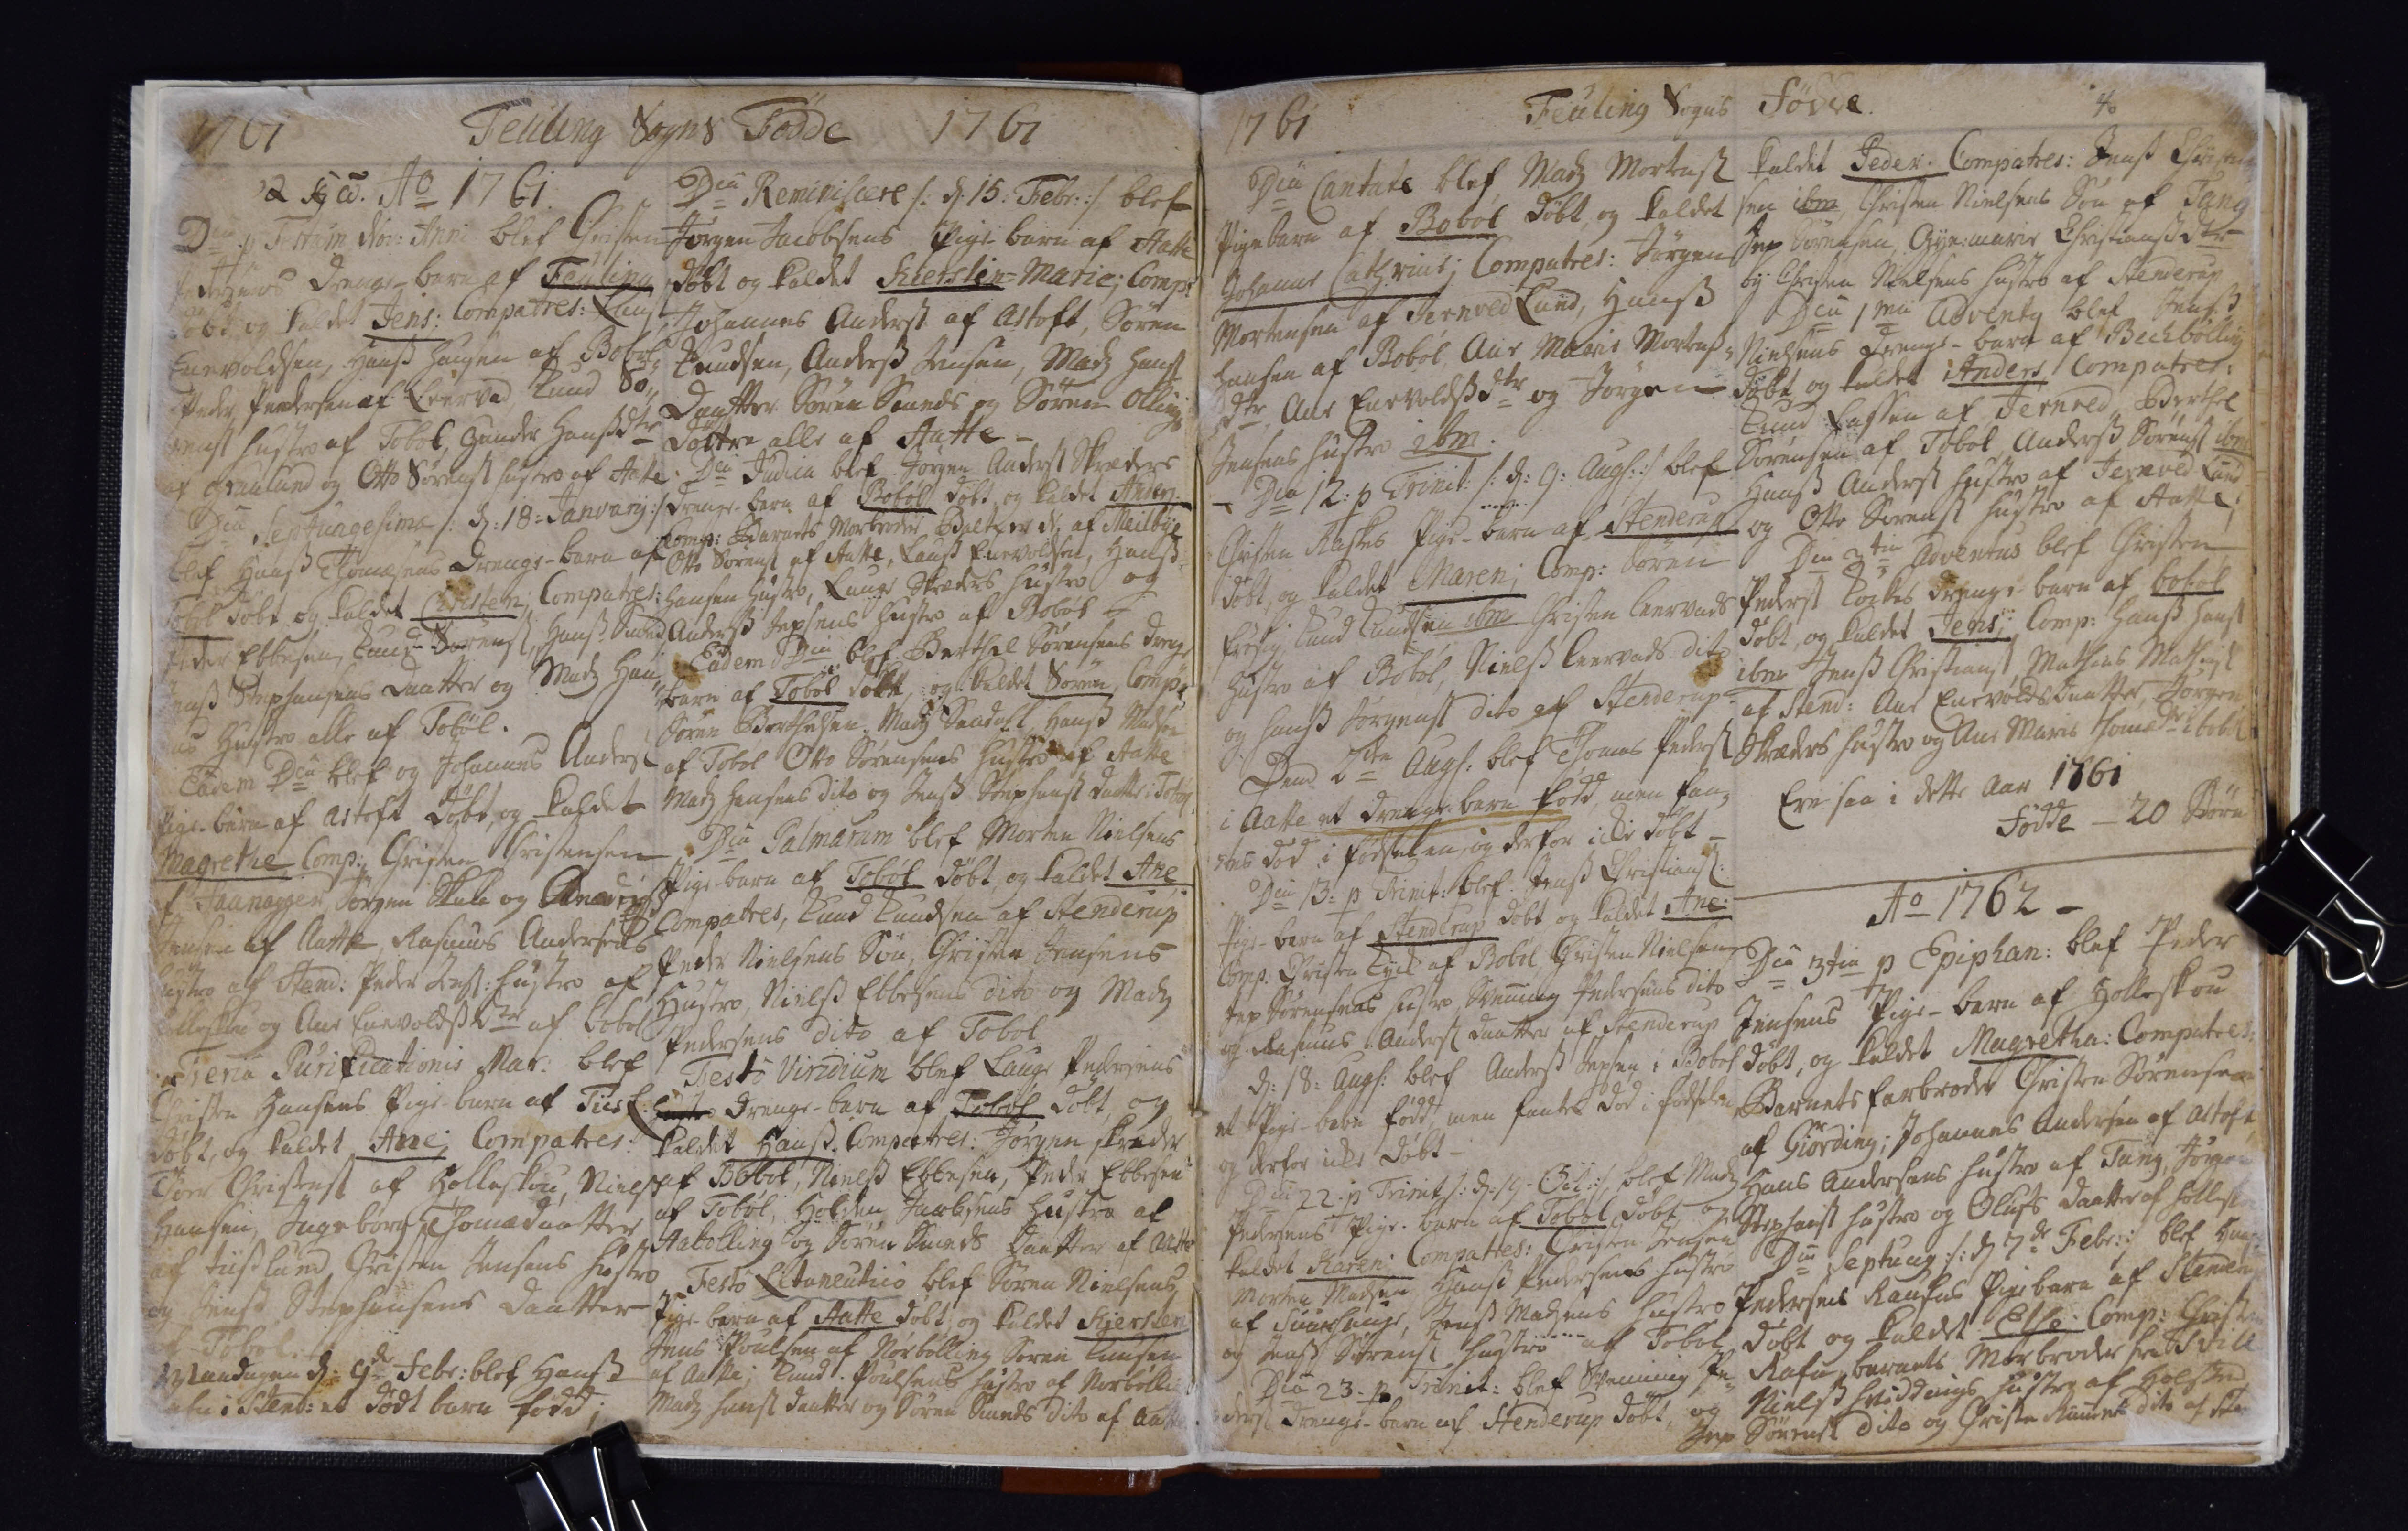1761
Feuling Sogns Fødde
##2 ##. Ao 1761
Dca p Festum Nov: Anni blef Christen
Pedersens Drenge-barn af Feuling
døbt og kaldet Jens; Compatres: Lau##
Enevoldsen, Hans Hansen af Bobøl
Peder Pedersen af Leervad, Knud Sø¬
sens Hustro af Tobøl, Gunder Hanssdtr
af Graulund og Otte Sørens Hustro af Aatte
Dca Septuagesima /: d: 18: Januarij :/
blef Hans Thomæsens Drenge-barn af
Tobøl døbt og kaldet Christen; Compatres:
Peder Ebbesøn, Knud Sørens Hans Smed
Jens Stephansens Daatter og Madz Han¬
sens Hustro alle af Tobøl.
Eodem Dca blef og Johannes Anders
Pige-barn af Aatoft døbt, og kaldet
Magrethe Comp:, Christen Christensen
af Faanagger Jørgen Skule og Anderss
Jensen af Aatte, Rasmus Andersens
Hustror af Stend: Peder Jens: Hustro af
Hollesakou og Ane Enevoldssdtr af bobøl
Feria Purificationis Mar: blef
Christen Hansens Pige-barn af Tiisl:
døbt, og kaldet Ane; Compatres:
Thoer Christens af Holleskou, Nielss
Hansen, Ingeborg Thomasdaatter
af Tiislund, Christen Jensens Hustro
og Jenss Stephansens Daatter,
af Tobøl
Mandagen d: 9de Febr: blef Hanss
Rafn i Stend: et dødt barn fødd;
1761
Dca Reminiscere /: d 15 Febr: :/ blef
Jørgen Jacobsens Pige-barn af Aatte
døbt og kaldet Kiersten=Marie; Comp:
Johannes Anders af Astoft, Søren
Knudsen, Anders Jensen, Madz Hans
Daatter. Søren Smeds og Søren Ollings
Døttre alle af Aatte
Dca Judica blef Jørgen Anders Skræders
Drenge-barn af Bobøl døbt, og kaldet Anders
Comp: Barnets Morbroder Baltzer N. af Meilbye
Otte Sørens af Aatte, Laus Envoldsen, Hanss
Hansen Hustro, Lauge Skræders Hustro og
Anderss Jepsens Hustro af Bobøl
Eadem Dca blef Berthel Sørensens Drenge¬
Barn af Tobøl døbt, og kaldet Søren, Comp:
Søren Berthelsen, Madz Sandahl, Hanss Madsen
af Tobøl Otto Sørrensens Hustro af Aatte
Matz Hansens dito og Jenss Stephans Daatter i Tobøl
Dca Palmarum blef Morten Nielsens
Pige-barn af Tobøl døbt, og kaldet Ane
Compatres, Knud Knudsen af Stenderup
Peder Nielsens Søn, Christen Jensens
Hustro, Nielss Ebbesens dito og Madz
Pedersens dito af Tobøl
Festo Viridium blef Lauge Pedersens
Hustro Drenge-barn af Tobøl døbt, og
kaldet Hanss, Compatres: Jørgen Skræder
af Bobøl, Nielss Ebbesen, Peder Ebbesen
af Tobøl, Holden Jacobsens Hustro af
Aabølling og Søren Smeds Daatter af Aatte
Festo Litaneutico blef Søren Nielsens
Pige barn af Aatte døbt, og kaldet Kiersten
Jens Poulsen af Nørbølling Søren Knudsen
af Aatte; Knud Povelsens Hustro af Nørbølling
Madz Hans daatter og Søren Smeds dito af Aatte
Feuling Sogns Fødde
1761
Dca Cantate blef Madz Mortens
Pigebarn af Bobøl døbt, og kaldet
Johanne Cathrine; Compatres: Jørgen
Mortensen af Jernved Lund, Hanss
Hansen af Bobøl, Ane Mrieads Mortens¬
dtr Ane Enevoldssdtr og Jørgen
Jensens Hustro ibm
Dca 12: p Trinit: /: d: 9: Augs: :/ blef
Christen Raskes Pige-barn af Stenderup
døbt, og kaldet Maren; Comp: Søren
Frøsig, Knud Knudsen ibm. Christen Leervads
Hustro af Bobøl, Nielss Leervads dito
og Hanss Jørgens dito af Stenderup
Dend 2den Augs: blef Thomas Peders
i Aatte et Drengebarn fødd, men fan¬
tes død i Førsten og derfor icke døbt-
Dca 13: p Trinit: blef Jenss Christians:
Pige-barn af Stenderup døbt og kaldet Ane
Comp: Christen Kyck af Bobøl Christen Nielsen
Jep Sørensens Hustro, Svenning Pedersens dito
og Rasmus Anders Daatter af Stenderup
d: 18: Augs: blef Anderss Jepsen i Bobøl
et Pige-barn fødd, men fantes død fødselen
og derfor icke døbt-
Dca 22 p Trinit: /: d: 19 Oct: :/ blef Madz
Pedersens Pige-barn af Tobøl døbt og
kaldet Karen Compatres: Christen Jensen
Morten Madsen, Hans Pedersens Hustro
af Suurhauge, Jens Madsens Hustro
og Jens Sørens Hustro af Tobøl
Dca 23 p. Trinit: bleff Svenning Pe¬
ders Drenge-barn af Stenderup døbt og
Jep
40
og kaldet Peder; Compatrer: Jens Christen¬
sen ibm, Christen Nielsens Søn af Tang
Jep Sørensen, Gye: Marie Christensdtr
og Christen Nielsens Hustro af Stenderup
Dca 1ma Advent## blef Jenss
Nielsens Drenge-barn af Bechbølling
døbt og kaldet Anders Compatres:
Knud Lassen af Jernved. Berthel
Sørensen af Tobøl Anders Sørens ibm
Hanss Anders Hustro af Jernved Lund
og Otto Sørens Hustro af Aatte;
Dca 3tia Adventus blef Christen
Peders Kockes Drenge-barn af bobøl
døbt, og kaldet Jens; Comp: Hanss Hans
ibm Jens Christians, Mathias Mathias
af Stend: Ane Envolds Daatter, Jørgen
Skræders Hustro og Ane Maris Thomædtr i bobøl
Ere saa i dette Aar 1761
Fødde - 20 Børn
Ao 1762-
Dca 3tia p Epiphan: blef Peder
Jensens Pige-barn af Holleskou
døbt, og kaldet Magretha: Compatres:
Barnets Farbroder Christen Sørensen
af Giording; Johannes Andersen af Astoft,
Hans Andersens Hustro af Tang, Jørgen
Stephans Hustro og Olufs Daatter af Holleskou
Dca Septuag: /: d 7de Febr: :/ bleff Hans
Pedersens Rauskes Pigebarn af Stenderup
døbt og kaldet Else; Comp: Christen
Rafn Barnets Morbroder fra Tvile
Niels Hviddings Hustro af Holsted
Sørens dito og Christen Ruue## dito af St##
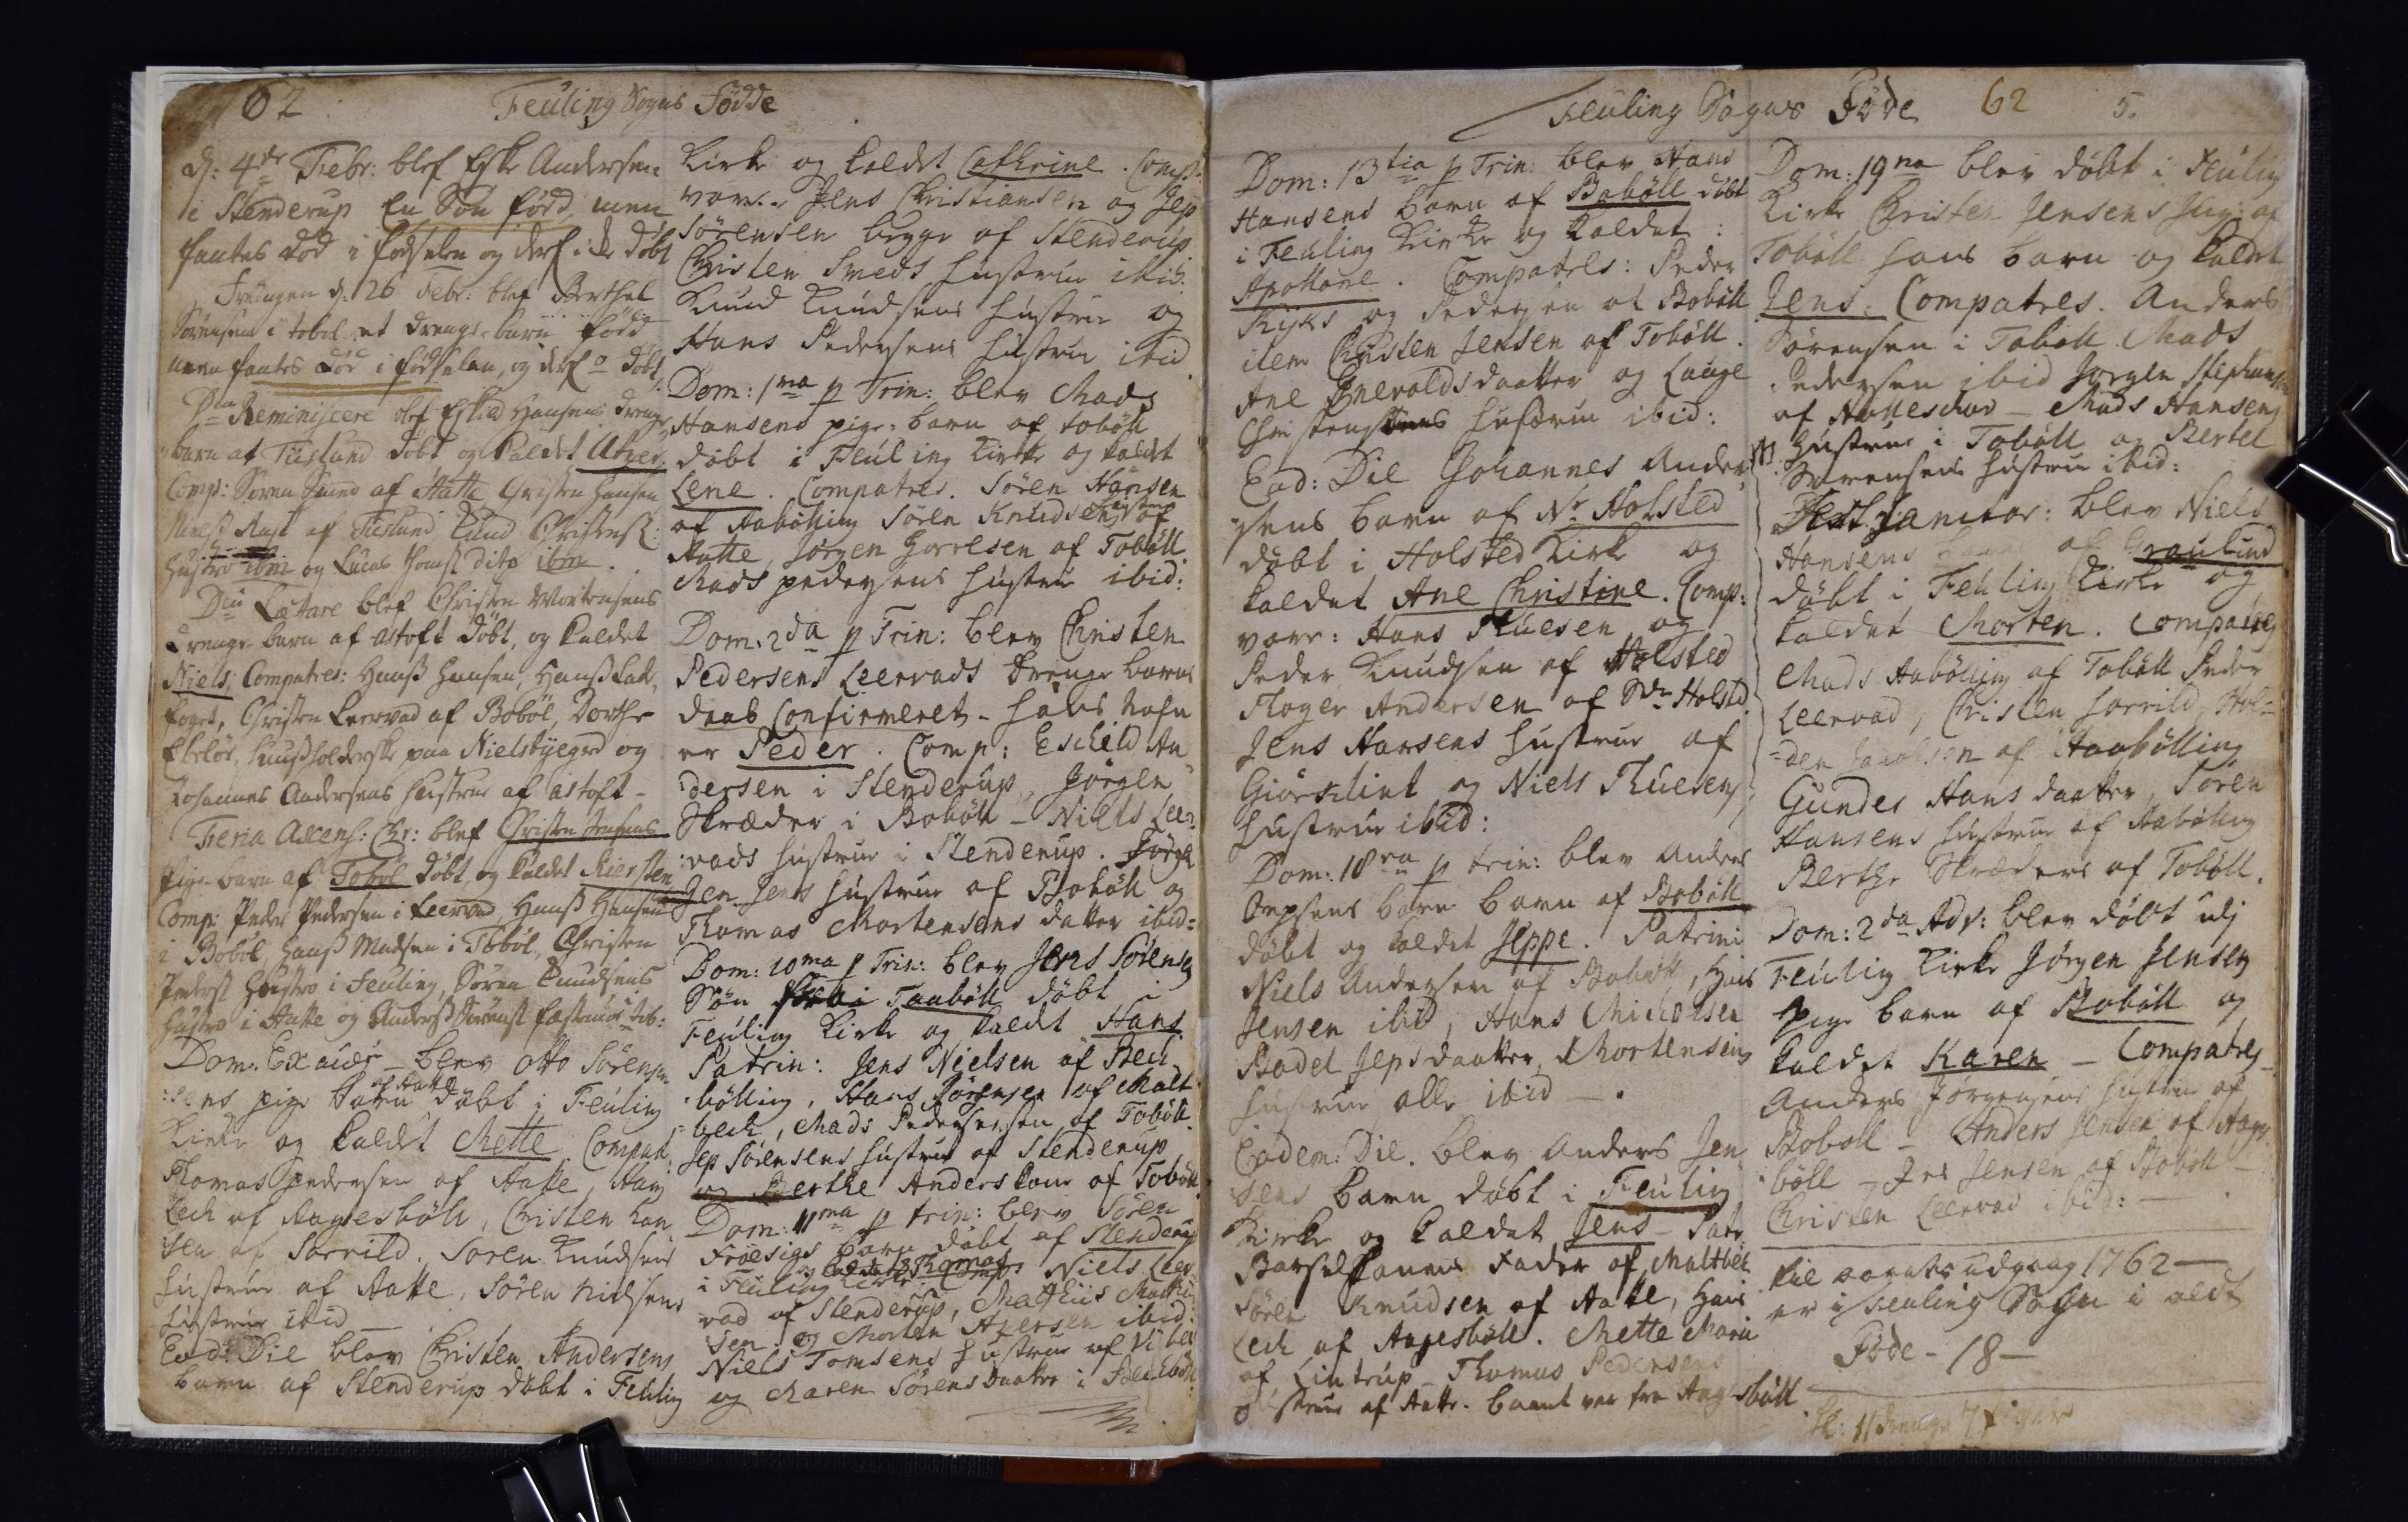1762
Feuling Sogns Fødde
d: 4de Febr: blef Eske Andersen
i Stenderup En Søn fødd, men
fantes død i Fødselen og derf icke døbt
Fredagen d: 26 Febr: blef Berthel
Sørensen i Tobøl et Drenge-barn fødd
men fantes død i Fødselen, og derf o døbt
Dca Reminiscere blef Eskild Hansens Drenge¬
= Barn af Tiislund døbt og kaldet Atzer,
Comp: Søren Smed af Aatte Christen Hansen
Niels Rask af Tiislund Knud Christens:
Hustro ibm og Lucas Thoms dito ibm
Dca Lætare blef Christen Mortensens
Drenge-barn af Astoft døbt, og kaldet
Niels; Compatres: Hanss Hanssen Hanss Lade¬
foget, Christen Leervad af Bobøl, Dorthe
Eleløv, Huussholderske paa Nielsbyegrd og
Johannes Andersens Hustrue af Astoft-
Feria Ascens: Chr: blef Christen Jensens
Pige-barn af Tobøl døbt, og kaldet Kiersten
Comp: Peder Pedersen i Leervad, Hans Hansen
i Bobøl, Hans Madsen i Tobøl, Christen
Peders Hustro i Feuling, Søren Knudsens
i
Hustro i Aatte og Anders Sørens Fæstemø ##tob:
Dom. Exaudi blev Otto Sørensen
af Aatte
sens pige barn døbt i Feuling
Kirke og kaldet Mette. Compat:
Thomas Pedersen af Aatte, Hans
Lech af Aagesbøll, Christen Lar¬
sen af Sorrild. Søren Knudsens
Hustrue af Aatte, Søren Nielsens
Hustrue ibid
Eod: Die blev Christen Andersens
Barn af Stenderup døbt i Feuling
Kirke og kaldet Cathrine. Comp.
vare.. Jens Christiansen og Jep
Sørensen begge af Stenderup
Christen Smeds Hustrue ibid.
Knud Knudsens Hustrue og
Hans Pedersens Hustru ibid
Dom: 1ma p Trin: blev Mads
Hansens pige=barn af Tobøl
døbt i Feuling Kirke og kaldet
Lene. Compatres. Søren Hansen
Hustrue
af Aabølling Søren Knudsens af
Aatte, Jørgen H##lsen af Tobøll
Mads Pedersens Hustru ibid
Dom: 2da p Trin: blev Christen
Pedersens Leervads Drenge barns
daab Confirmeret Hans Nafn
er Peder. Comp: Eschild An¬
derssen i Stenderup, Jørgen
Skræder i Bobøll - Niels Leer¬
vads Hustrue i Stenderup. Jørgen
gen sens Hustrue af Tobøll og
Thomas Mortensens datter ibid:
Dom: 10ma p Trin: blev Jens Sørensens
Søn ## i Taabøll døbt i
Feuling Kirke og kaldet Hans.
Patrin: Jens Nielsen af Bech¬
= bølling, Hans Sørensen af Malt¬
= bech, Mads Pedersensen af Tobøll
Jep Sørensens Hustrue af Stenderup
og Berthel Anders Kone af Tobøll
Dom: 11ma p Trin: blev Søren
Frøesigs barn døbt af Stenderup
og kaldet Thomas
i Feuling Kirke. Comp: Niels Leer¬
vad af Stenderup, Mathiis Mathis¬
sen og Morten Atzersen ibid:
Niels Tomsens Hustrue af Vi##lev
og Maren Sørens daatter i F##bøll
Feuling Sogns Føde
Dom: 13tia p Trin: blev Hans
Hansens Barn af Bobøll døbt
i Feuling Kirke og kaldet:
Apollone. Compatres: Peder
Fyks og Pedersen af Bobøll
item Christen Jensen af Tobøll.
Ane Enevoldsdaatter og Lauge
Christensens Hustrue ibid:
Eod: Die Johannes Ander¬
sens barn af Nr Holsted
døbt i Holsted Kirke og
kaldet Ane Christine. Comp:
vare: Hans Thuesen og
Peder Knudsen af Holsted
Thoger Andersen af Sdr Holsted
Jens Hansens Hustrue af
Giørklint og Niels Thuesens
Hustrue ibid:
Dom: 18na p Trin: blev Anders
Jepsens barn barn af ##bøll
døbt og kaldet Jeppe. Patrini
Niels Andersen af Bobøll, Hans
Jensen ibid: Hans Michelsen
Bodel Jepsdaatter, Mortensens
Hustrue alle ibid.
Eodem: Die blev Anders Jens¬
sens barn døbt i Feuling
Kirke og kaldet Jens. Patr:
Barselkonens Fader af Maltbek
Søren Knudsen af Aatte, Hans
Ldch af Aagesbøll. Mette Marie
af Lintrup - Thomas Pedersens
Hustrue af Aatte. Barnet var fra Aagesbøll
5.
62.
Dom: 19na blev døbt i Feuling
Kirke Christen Jensens  Jun: af
Tobøll hans Barn og kaldet
Jens: Compatres: Anders
Sørensen i Tobøll. Mads
Pedersen ibid Jørgen Stephans
af Holleschov - Mads Hansens
Hustrue i STobøll og Bertel
Sørensens Hustru ibid:
Fest. Janitor: blev Niels
Hansens barn af Graulund
døbt i Feuling Kirke og
kaldet Morten. Compatres
Mads Aabølling af Tobøll Peder
Leervad, Christen Sorrild, Hol¬
= den Jacobsen af Aaabølling
Gunder Hans Daatter, Søren
Hansens Hustrue af Aabølling
Berthe Skræder af Tobøl.
Dom: 2da Adv: blev døbt udj
Feuling Kirke Jørgen Jensens
Pige Barn af Bobøll og
kaldet Karen - Compatres
Anders Jørgensens Hustrue af
Bobøll - Anders Jensen af Aages¬
bølle - Jes Jensen af Bobøll
Christen Leervad ibid: -
til aarets udgang 1762 -
eri Feuling Sogn i aldt
Føde - 18 -
##: 11 Drenge 7 Piger